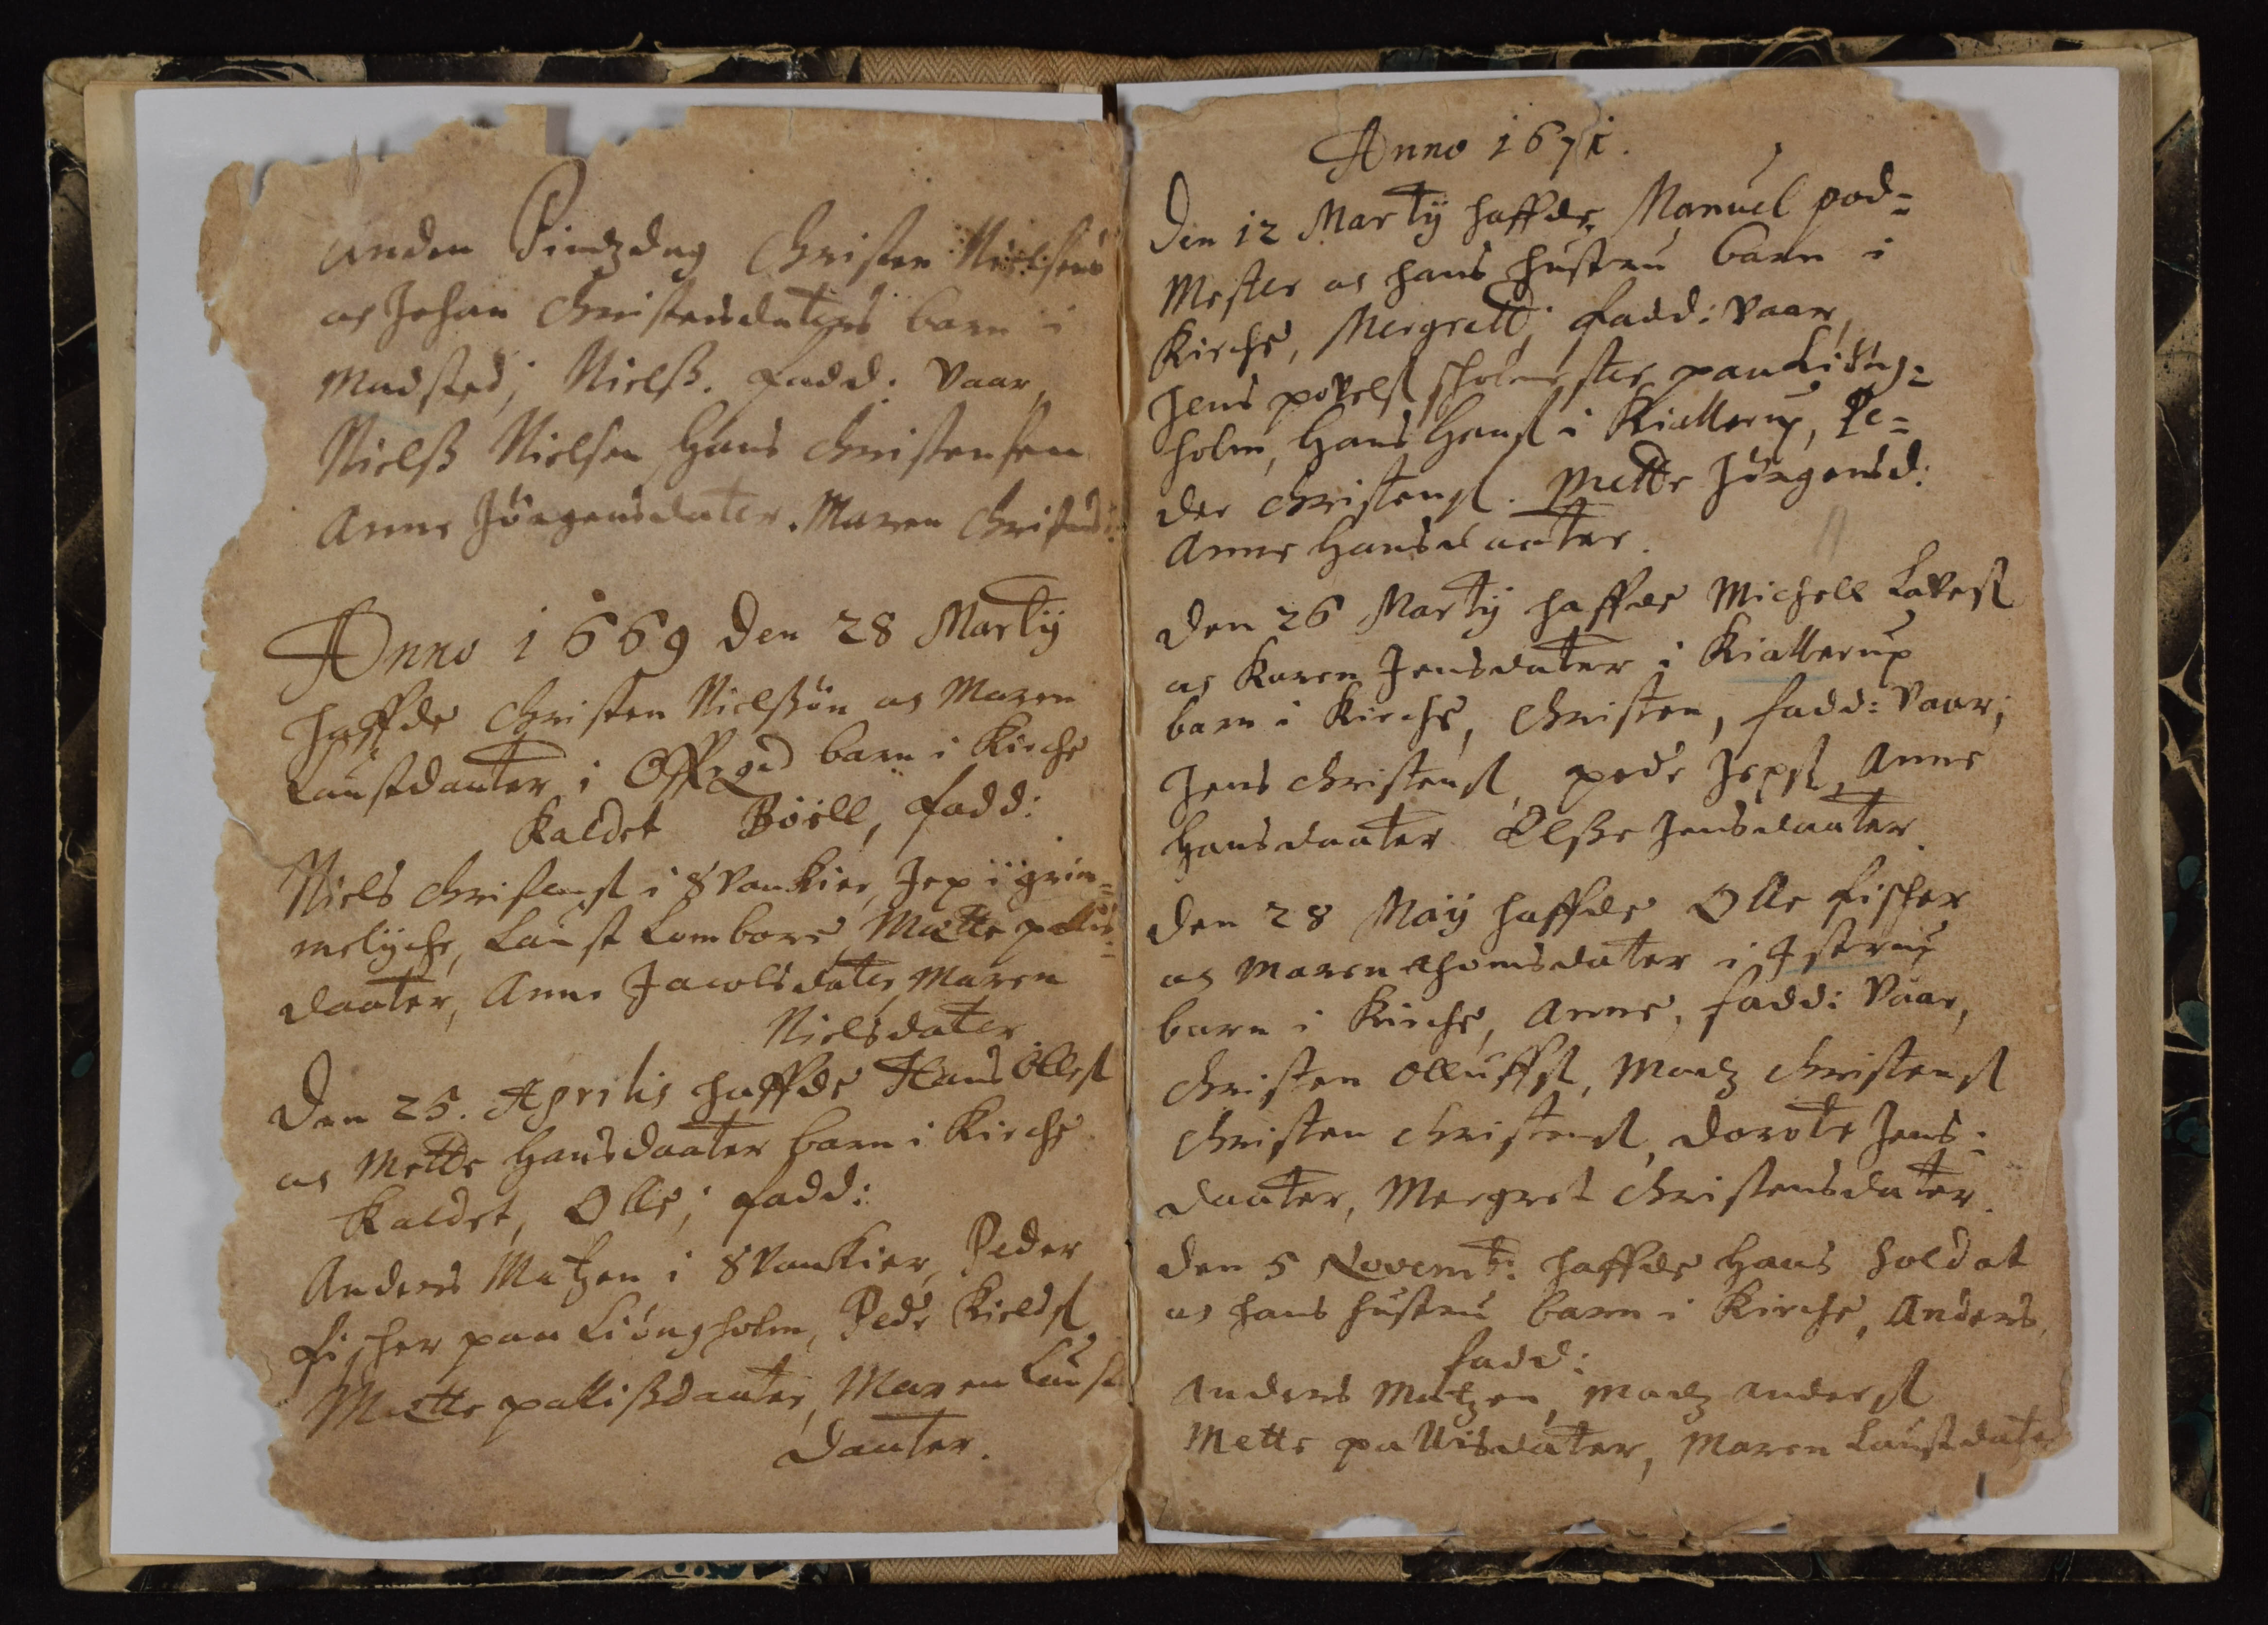Anden Pindzdag Christen Nielsens
och Johan Christensdaters barn i
Madsted; Nielss Fadd: vaar
Nielss Nielsen Hans Christensen
Anne Jørgensdater Maren Christensd:
Anno 1669 den 28 Martij
haffde Christen Nielssøn och Maren
Laustdauter i Offegrd barn i Kirche
kaldet Boell, Fadd:
Niels Christens i Svankier Jep i Grim¬
melyche, Laust Lombore Mætte pallis¬
daater, Anne Jacobsdater Maren
Nielsdater
Den 25. Aprilis haffde Fans Olles
och Mette Hansdaater barn i Kirche
kaldet, Olle, Fadd:
Anders Matzen i Svankier Peder
Ficher paa Liungholm, Pedr Kields
Meette pallisdauter Mare en Laust
dauter
Anno 1671.
Den 12 Martij haffde Manuel pod¬
Mester och hans hustruu barn i
Kirche, Mergrett Fadd: vaar
Jens povels sholemester paa Liung¬
holm, Hans Hans i Kiallerup, Pe¬
der Christens. Mette Jørgensd:
Anne Hansdaater
Den 26 Martij haffde Michell Lavest
och Karen Jensdater i Kiallerup
barn i Kirche, Christen, Fadd: vaar
Jens Christens pedr Jeps Anne
Hansdaater Elsse Jensdaater
den 28 Maij haffde Olle Fisher
och Maren Thomsdater i Istrup
barn i Kirche, Anne, fadd: vaar
Christen Olluffs, Madz Christens
Christen Christens Dorote Jens¬
daater, Mergret Christensdater
den 5 Novemb: haffde Hans soldat
och hans hustru barn i Kirche, Anders
Fadd:
Anders Matzen, Madz Anders
Mette pallisdatter, Maren Lausdater
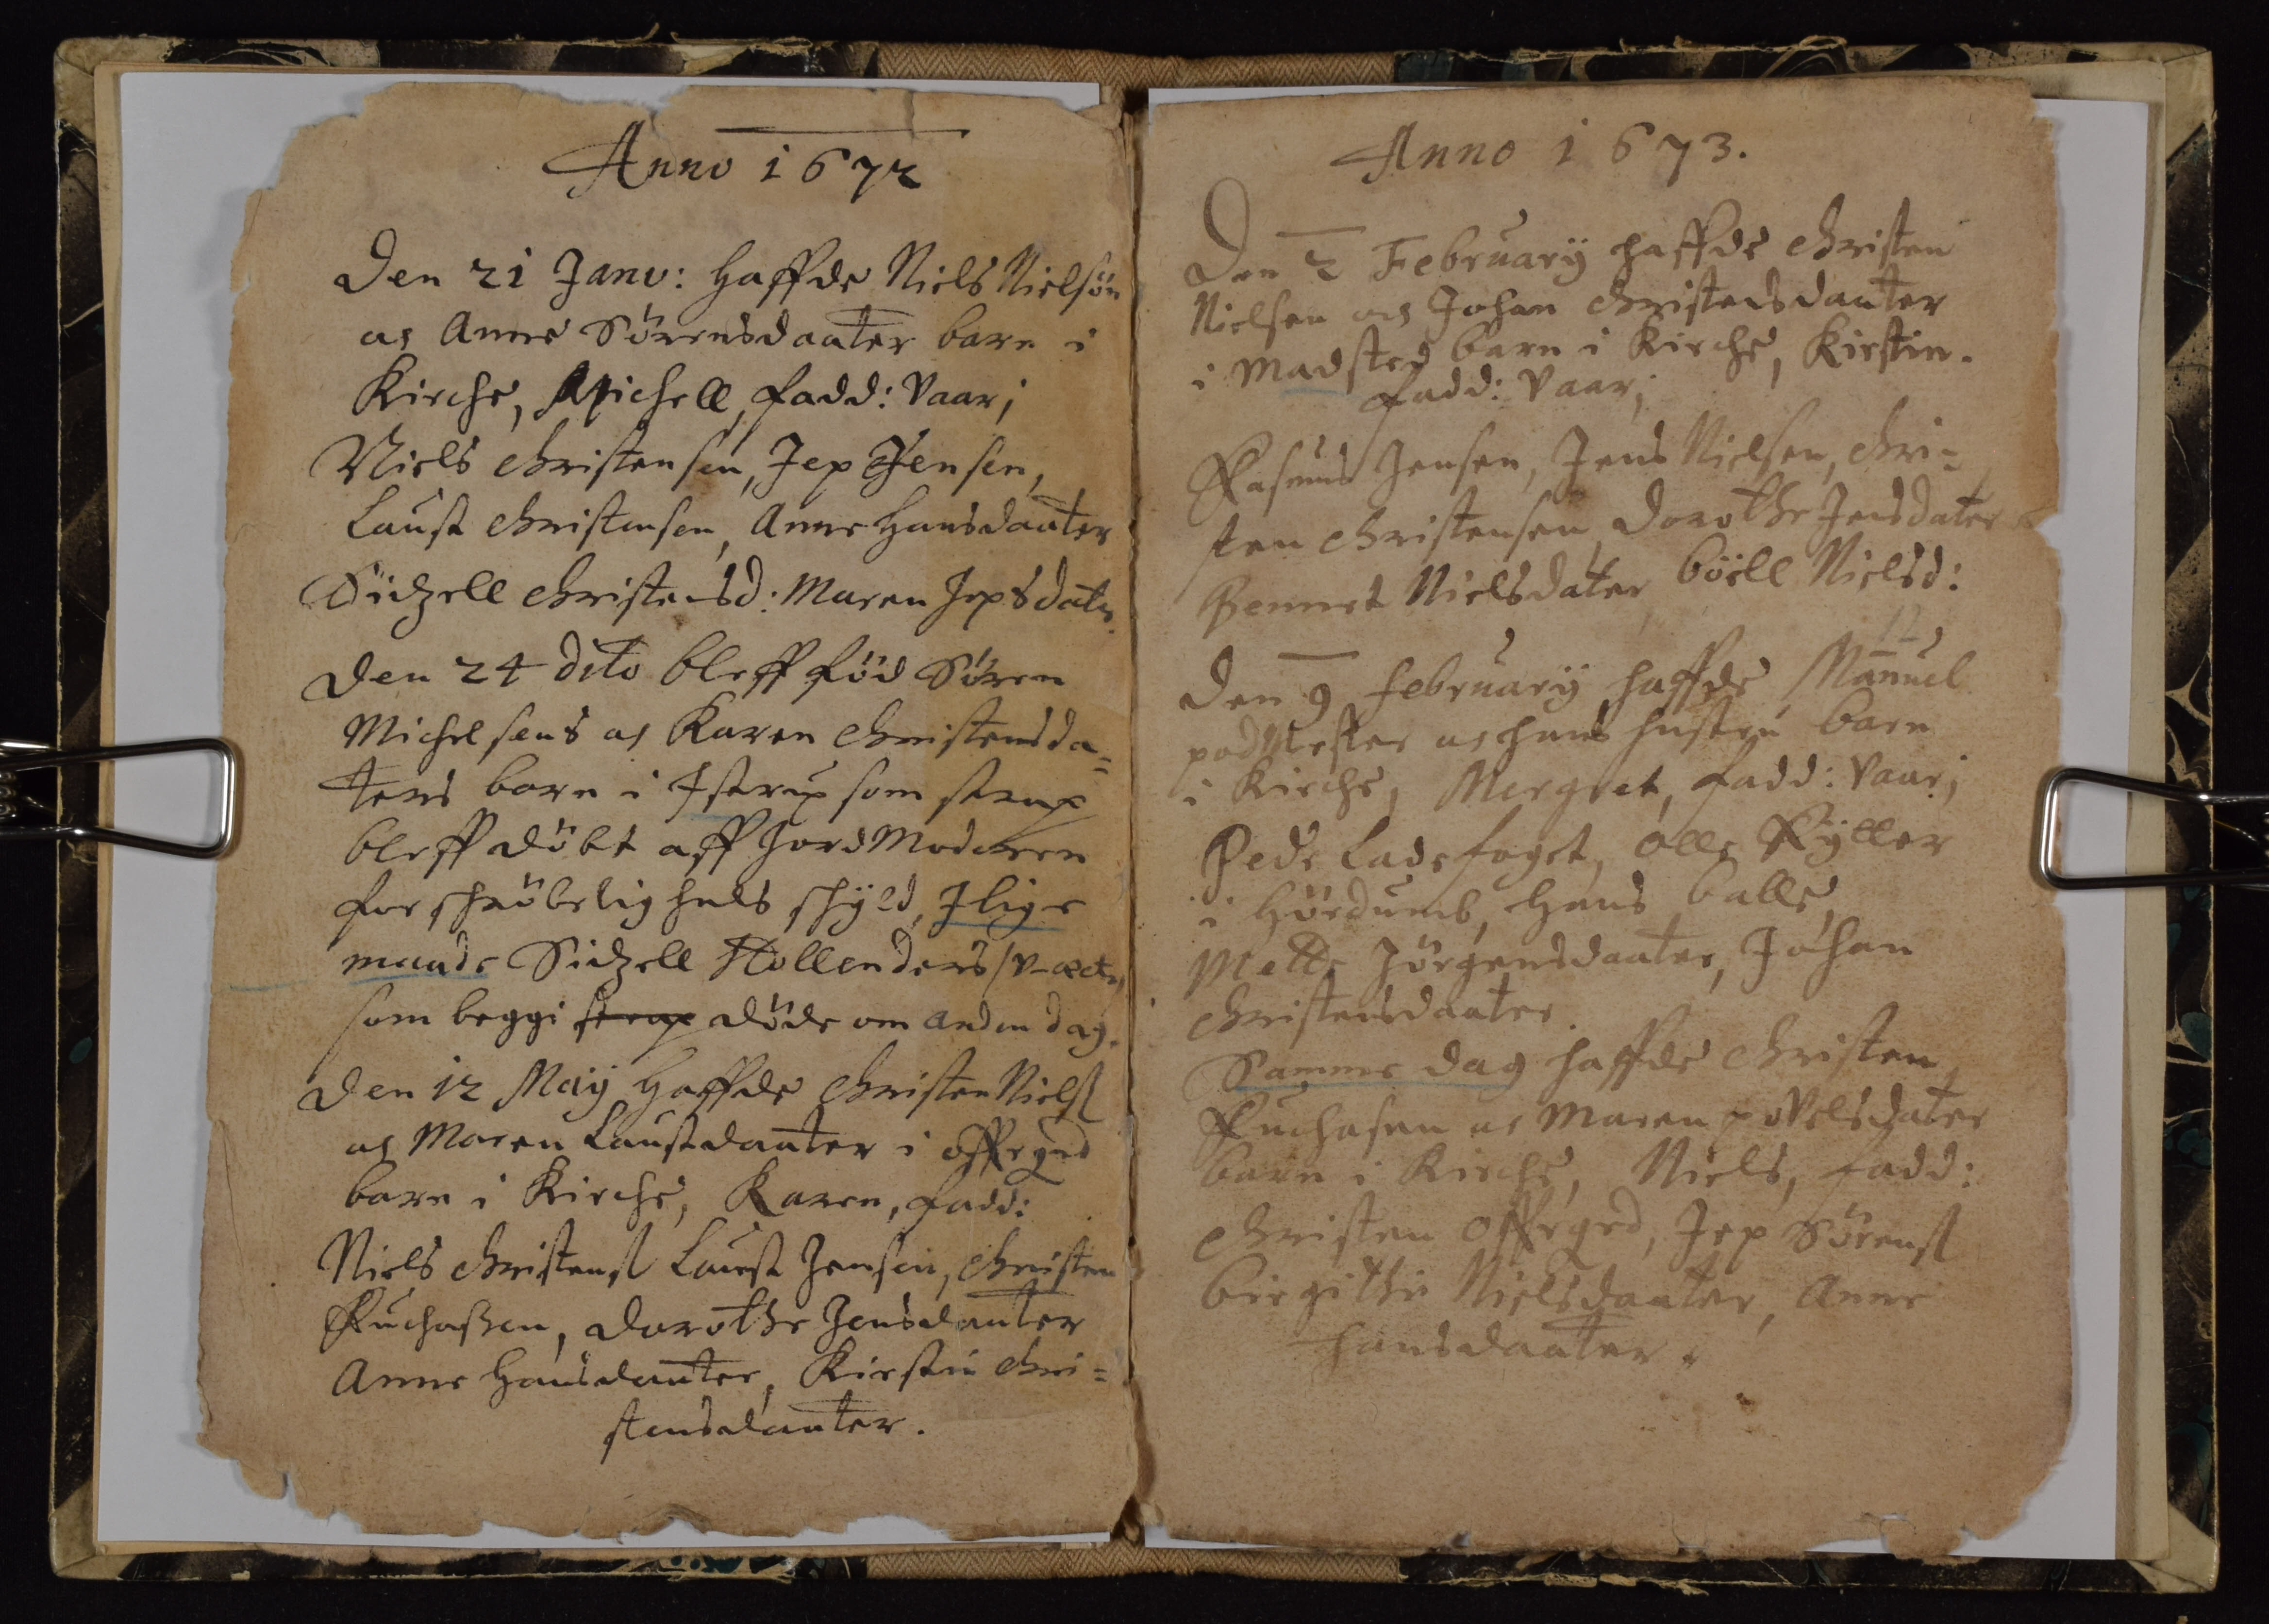Anno 1672
Den 21 Janu: Haffde Niels Nielsøn
och Anne Sørensdaater barn i
Kirche Michell Fadd: vaar;
Niels christensen, Jep Jensen,
Laust christensen Anne Hansdauter
Sidzell christensd: Maren Jepsdater
Den 24 dito bleff fød Søren
Michelsens och Karen christensda¬
ters born i Istrup som staax
bleff døbt aff Jord Moderen
for shrøbelig hels shyld, Ilige
maade Sidzell Hollenders / v-æcte
som begge strax døde om anden dag.
Den 12 Maij Haffde Christen Niels
och Maren Laustdauter. offegrd
barn i Kirche, Karen, Fadd:
Niels Christens Laust Jensen, Christen
Luchassen, Dorothe Jensdauter
Anne Hansdauter, Kirstin chri¬
stensdauter.
Anno 1673.
Den 2 Februarij haffde Christen
Nielsen och Johan Christensdauter
i Madsted barn i Kirche, Kirstin.
Fadd: vaar
Rasmus Jensen, Jens Nielsen chri¬
sten christensen dorothe Jensdater
Bennet Nielsdater boell Nielsd:
Den 9 Februarij haffde Mannuel
podmester och hans hustru barn
i Kirche, Mergret, Fadd: vaar
Pedr Ladefoget Olle Rytter
i Hørdumb Hans balle,
Mette Jørgensdaater, Johan
Christensdaater
Samme dag haffde Christen
Luchasen och Maren povelsdater
barn Kirche, Niels: Fadd:
Christen Offegrd, Jep Sørens
Birgithe Nielsdaater Anne
Hansdaater